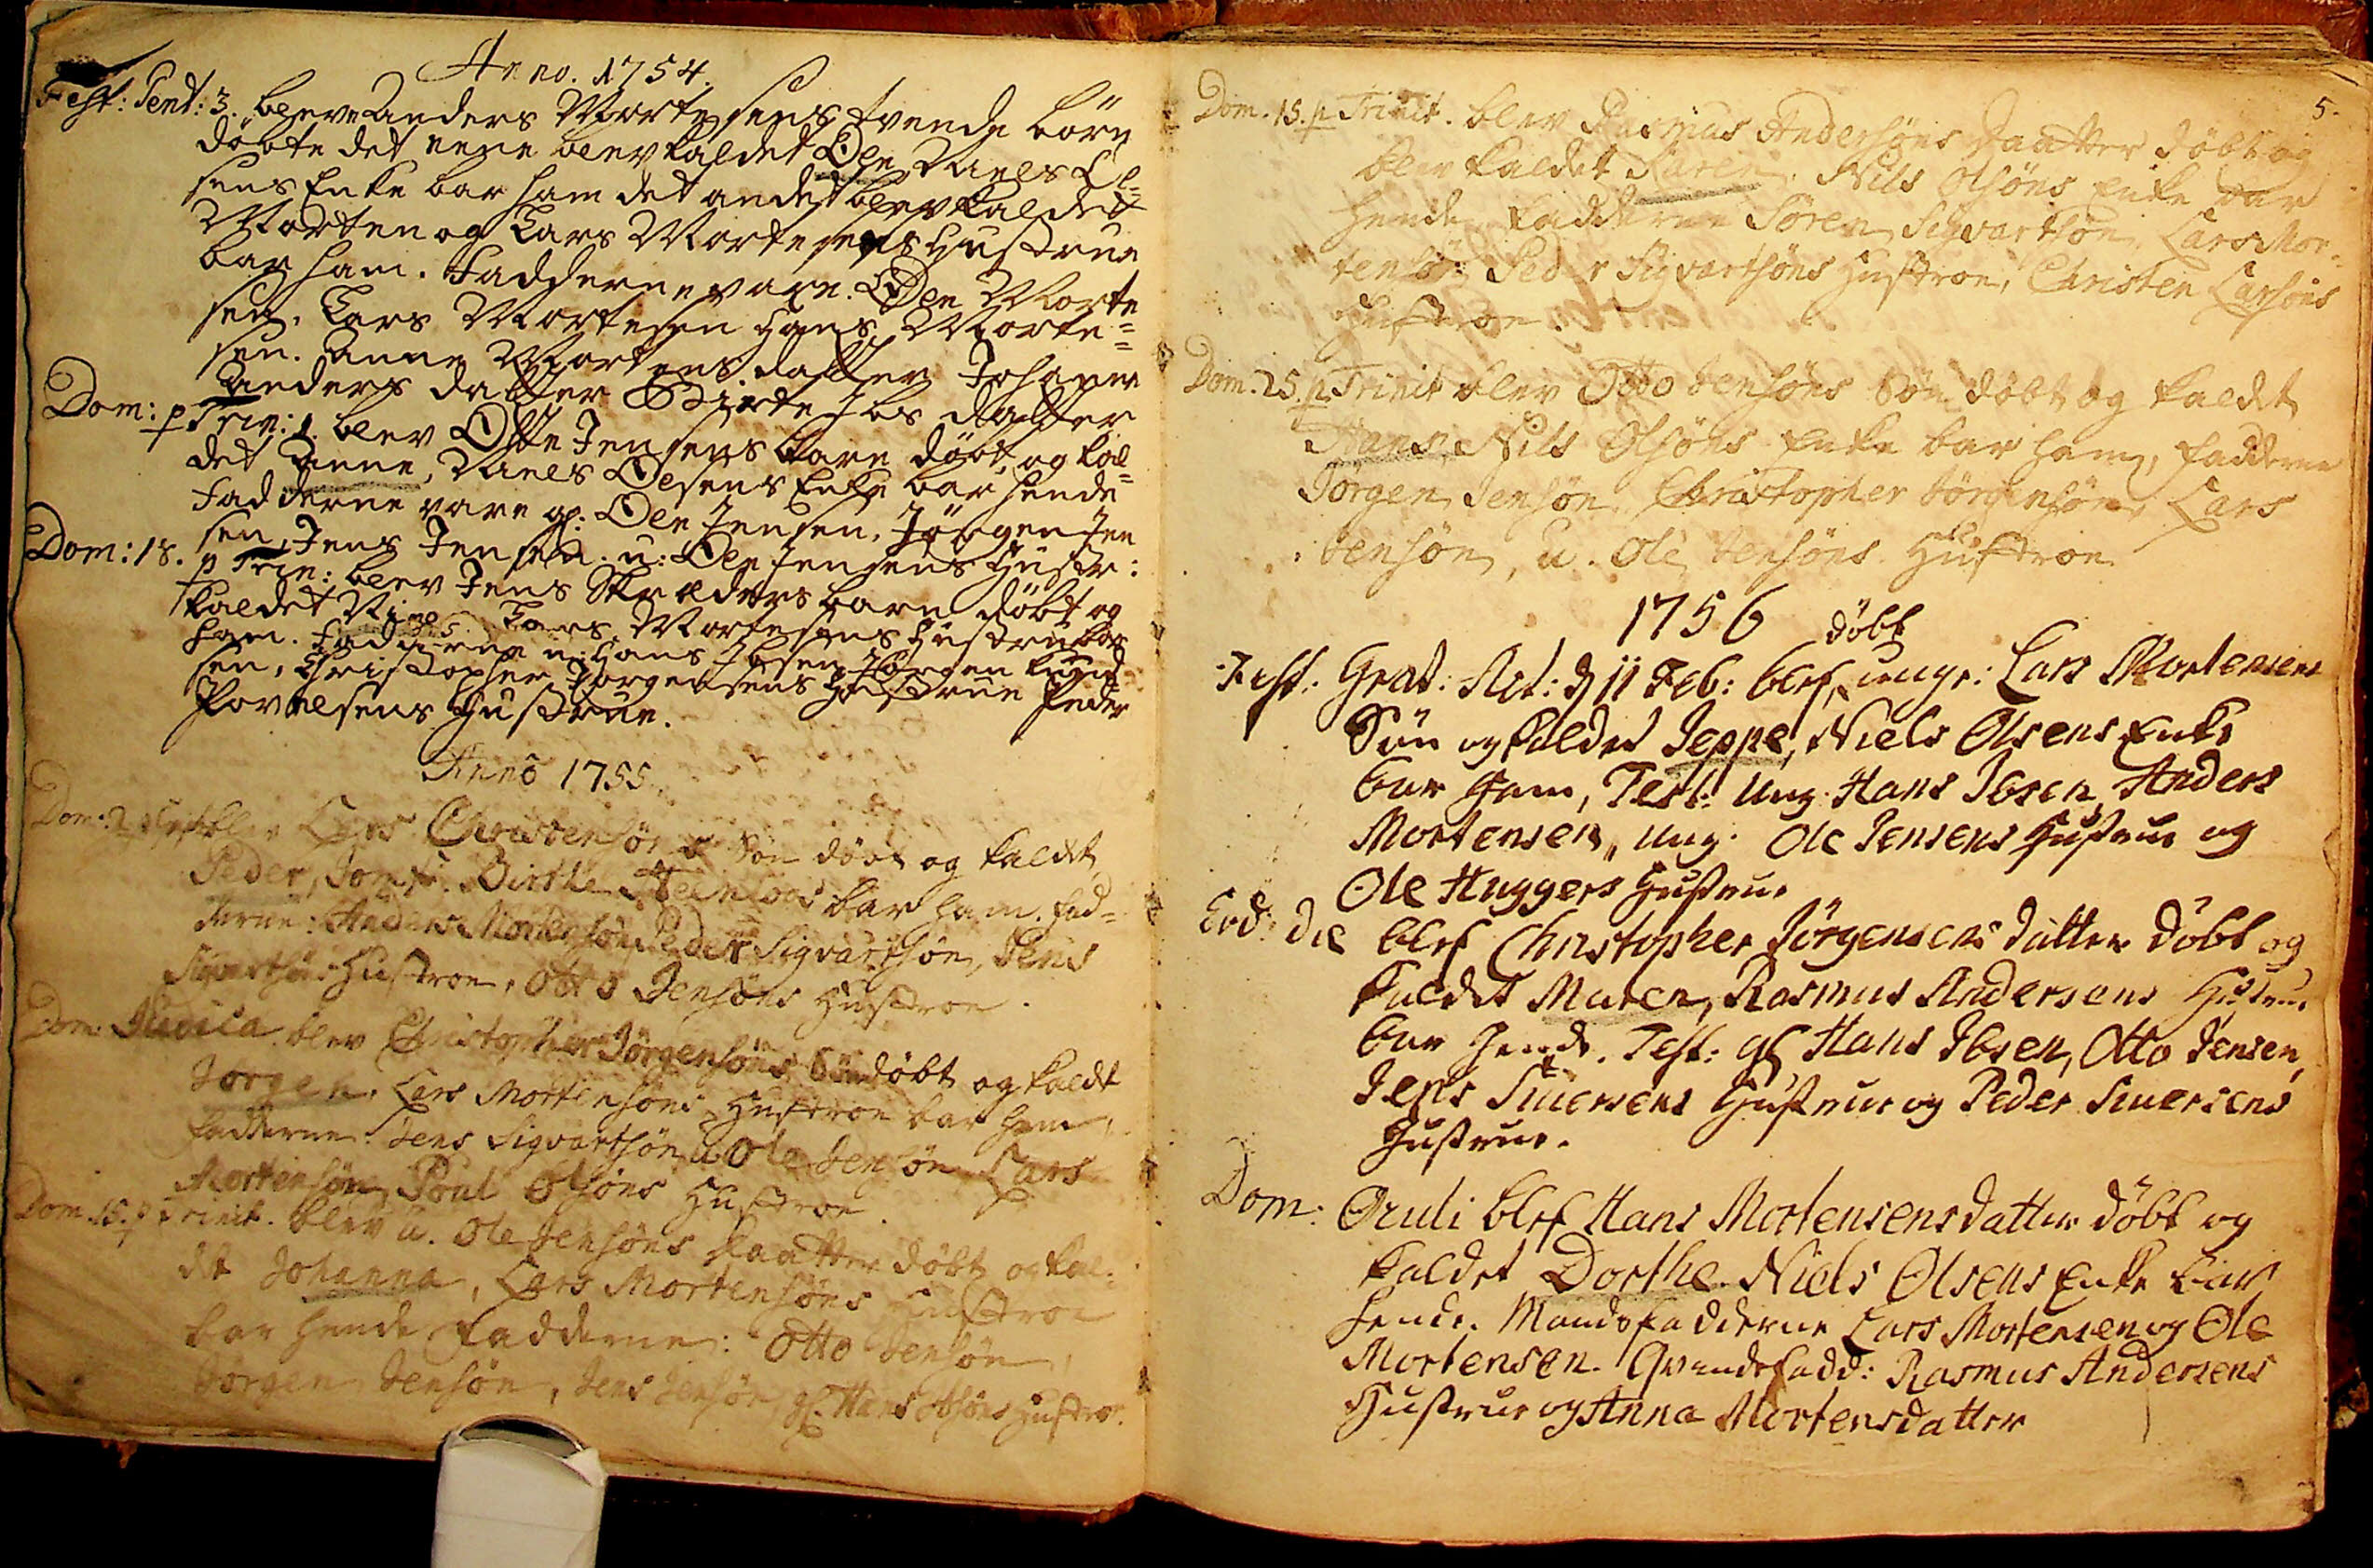Anno 1754.
Fest: Pent: 3. bleve Anders Mortensens tvende børn
døbte det eene blev kaldet Ole Niels Ol¬
sens Enke bar ham det andet blev kaldet
Morten og Lars Mortensens Hustrue
bar ham. Fadderne vare. Ole Morten¬
sen. Lars Mortensen Hans Morten¬
sen. Anne Mortensdatter Johanne
Anders datter Birte Ibs datter
Dom: p Trin: 1 blev Otte Jensens barn døbt og kal¬
det Anne. Niels Olsens Enke bar hende
Fadderne vare gl: Ole Jensen, Jørgen Jen
sen, Jens Jensen. u: Ole Jensens Hustr:
Dom: 18. p Trin: blev Jens Skræders barn døbt og
kaldet Niels. Lars Mortensens Hustrue bar
ham. Fadderne u: Hans Ibsen Jørgen Knud=
sen. Christopher Jørgensens Hustrue Peder
Povelsens Hustrue.
Anno 1755.
Dom: 2. p Epiph blev Lars Christensøns Søn døbt og kaldet
Peder, Jomfr. Birthe Steenloos bar ham. Fad¬
derne: Anders Mortensøn, Peder Sigvartsøn, Jens
Sigvartsøns Hustroe, Otto Jensøns Hustroe.
Dom: Judica blev Christopfer Jørgensøns Søn døbt og kaldt
Jørgen. Lars Mortensøns Hustroe bar ham,
Fadderne: Jens Sigvartsøn u. Ole Jensøn, Lars
Mortensøn, Poul Olsens Hustroe.
Dom. 15. p Trinit. blev u. Ole Jensøns Daatter døbt og kal¬
dt Johanna, Lars Mortensøns Hustroe
bar hende, Fadderne: Otto Jensøn,
Jørgen Jensøn, Jens Jensøn, gl Hans Ibsøns Hustroe.
5.
Dom 15. p Trinit. blev Rasmus Andersøns Daatter døbt og
blev kaldet Karen. Nils Olsøns Enke bar
hende, Fadderne Søren Sigvartsøn, Lars Mor¬
tensøn, Peder Sigvartsøns Hustroe, Christen Larsøns
Hustroe.
Dom. 25. p Trinit blev Otto Jensøns Søn døbt og kaldt
Hans, Nils Olsøns Enke bar ham, Fadderne
Jørgen Jensøn, Christopfer Jørgensøn, Lars
Jensøn, u. Ole Jensøns Hustroe.
1756 døbt
Fest: Grat: Act: d 11 Feb: blef, unge: Lars Mortensens
Søn og Kaldet Jeppe, Niels Olsens Enke
bar ham, Test: Ung. Hans Ibsen, Anders
Mortensen, Ung. Ole Jensens Hustrue og
Ole Huggers Hustrue.
Eod die blef Christopfer Jørgensens datter Døbt og
kaldet Maren, Rasmus Andersens Hustrue
bar hende. Test: gl Hans Ibsen, Otto Jensen,
Jens Siversens Hustrue og Peder Siversens
Hustrue.
Dom: Oculi blef Hans Mortensens datter døbt og
kaldet Dorthe. Niels Olsens Enke bar
hende, Mandfadderne  Lars Mortensen og Ole
Mortensen. Qvindefadd: Rasmus Andersens
Hustrue og Anna Mortensdatter  
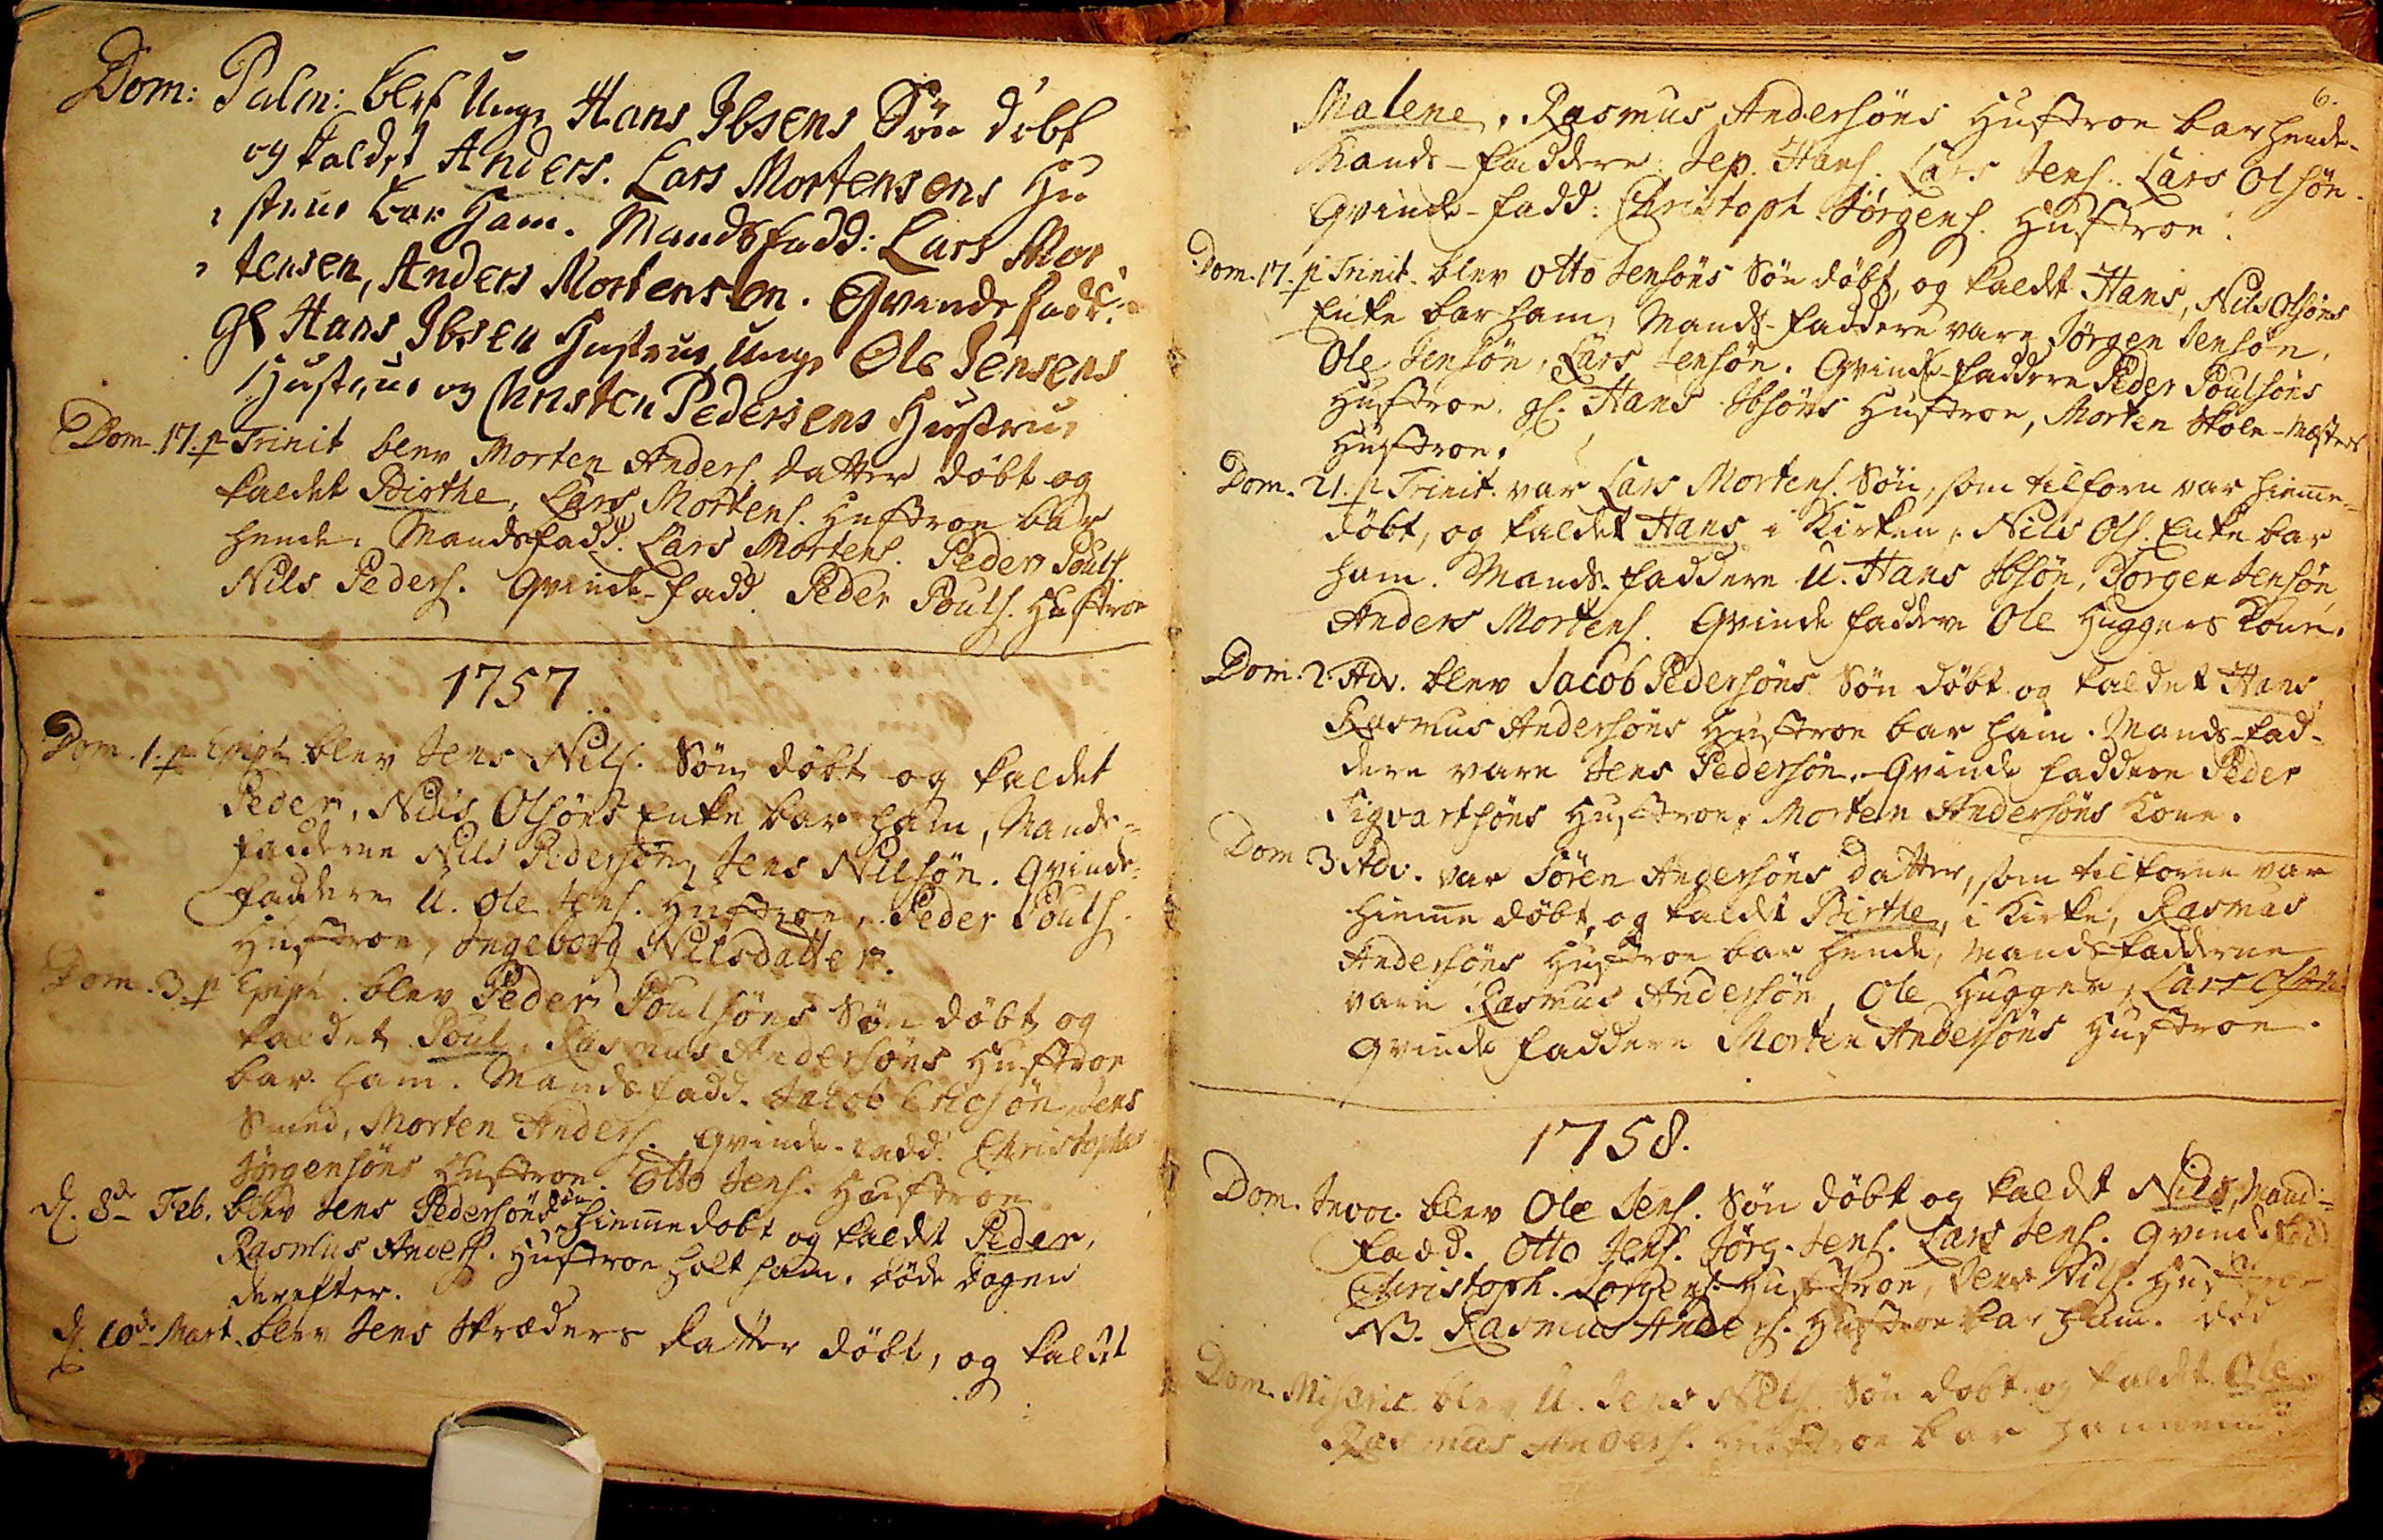Dom: Palm: blef Unge Hans Ibsens Søn døbt
og kaldet Anders. Lars Mortensens Hu
strue bar Ham. Mandfadd: Lars Mor
tensen, Anders Mortensen. Qvindefadd:
gl Hans Ibsen Hustrue, Unge Ole Jensens
Hustrue og Christen Pedersens Hustrue.
Dom. 17. p Trinit blev Morten Anders. Datter døbt og
kaldet Birthe, Lars Mortens. Hustroe bar
hende. Mandfadd. Lars Mortens. Peder Pouls.
Nils Peders. Qvinde-fadd Peder Pouls. Hustroe
1757
Dom. 1. p Epiph. blev Jens Nils. Søn døbt og kaldet
Peder, Nils Olsøns Enke bar ham, Mands¬
fadderne Nils Pedersøn, Jens Nilsøn. Qvinde¬
fadderne U. Ole Jens. Hustroe. Peder Pouls.
Hustroe, Ingeborg Nilsdatter.
Dom. 3. p Epiph. blev Peder Poulsøns Søn døbt og
kaldet Poul. Rasmus Andersøns Hustroe
bar ham. Mandsfadd. Jacob Ericsøn, Jens
Smed, Morten Anders. Qvinde-fadd. Christopher
Jørgensøns Hustroe. Otto Jens. Hustroe.
d. 8de Feb. blev Jens Pedersøns Søn hiemedøbt og kaldt Peder,
Rasmus Anders. Hustroe holt ham. Døbt Dagen
derefter.
d. 10de Mart. blev Jens Skræders datter døbt og kaldt
6.
Malene. Rasmus Andersøns Hustroe bar hende.
Mands-faddere. Jep. Hans. Lars Jens. Lars Olsøn
Qvinde-fadd. Christoph. Jørgens. Hustroe.
Dom. 17. p Trinit blev Otto Jensøns Søn døbt, og kaldet Hans, Nils Olsøns
Enke bar ham, Mands-faddere vare Jørgen Jensøn,
Ole Jensøn, Lars Jensøn. Qvinde-faddere Peder Poulsøns
Hustroe. gl. Hans Ibsøns Hustroe, Morten Skole-Mæsters
Hustroe.
Dom. 21. p Trinit. var Lars Mortens. Søn, som tilforn var hieme¬
døbt, og kaldet Hans i Kirken, Nils Ols. Enke bar
ham. Mands-faddere U. Hans Ibsøn, Jørgen Jensøn,
Anders Mortens. Qvinde faddere Ole Huggers Kone.
Dom. 2. Adv. blev Jacob Pedersøns Søn døbt og kaldet Hans,
Rasnus Andersøns Hustroe bar ham. Mands-fad¬
dere vare Jens Pedersøn. Qvinde faddere Peder
Sigvartsøns Hustroe, Morten Andersøns Kone.
Dom 3 Adv. var Søren Andersøns datter, som tilforen var
hieme døbt, og kaldt Birthe, i Kirke, Rasmus
Andersøns Hustroe bar hende, Mands-Fadderne
vare Rasmus Andersøn, Ole Hugger, Lars Olsøn
Qvinde faddere Morten Andersøns Hustroe.
1758.
Dom. Invoc. blev Ole Jens. Søn døbt og kaldt Nils, Mand¬
fadd. Otto Jens. Jørg. Jens. Lars Jens. Qvindefadd.
Christoph. Jørgens. Hustroe, Jens Nils. Hustroe
NB. Rasmus Anders. Hustroe bar ham. død
Dom. Miseric, blev U. Jens Nils. Søn døbt og kaldet Ole
Rasmus Anders. Hustroe bar hannem.